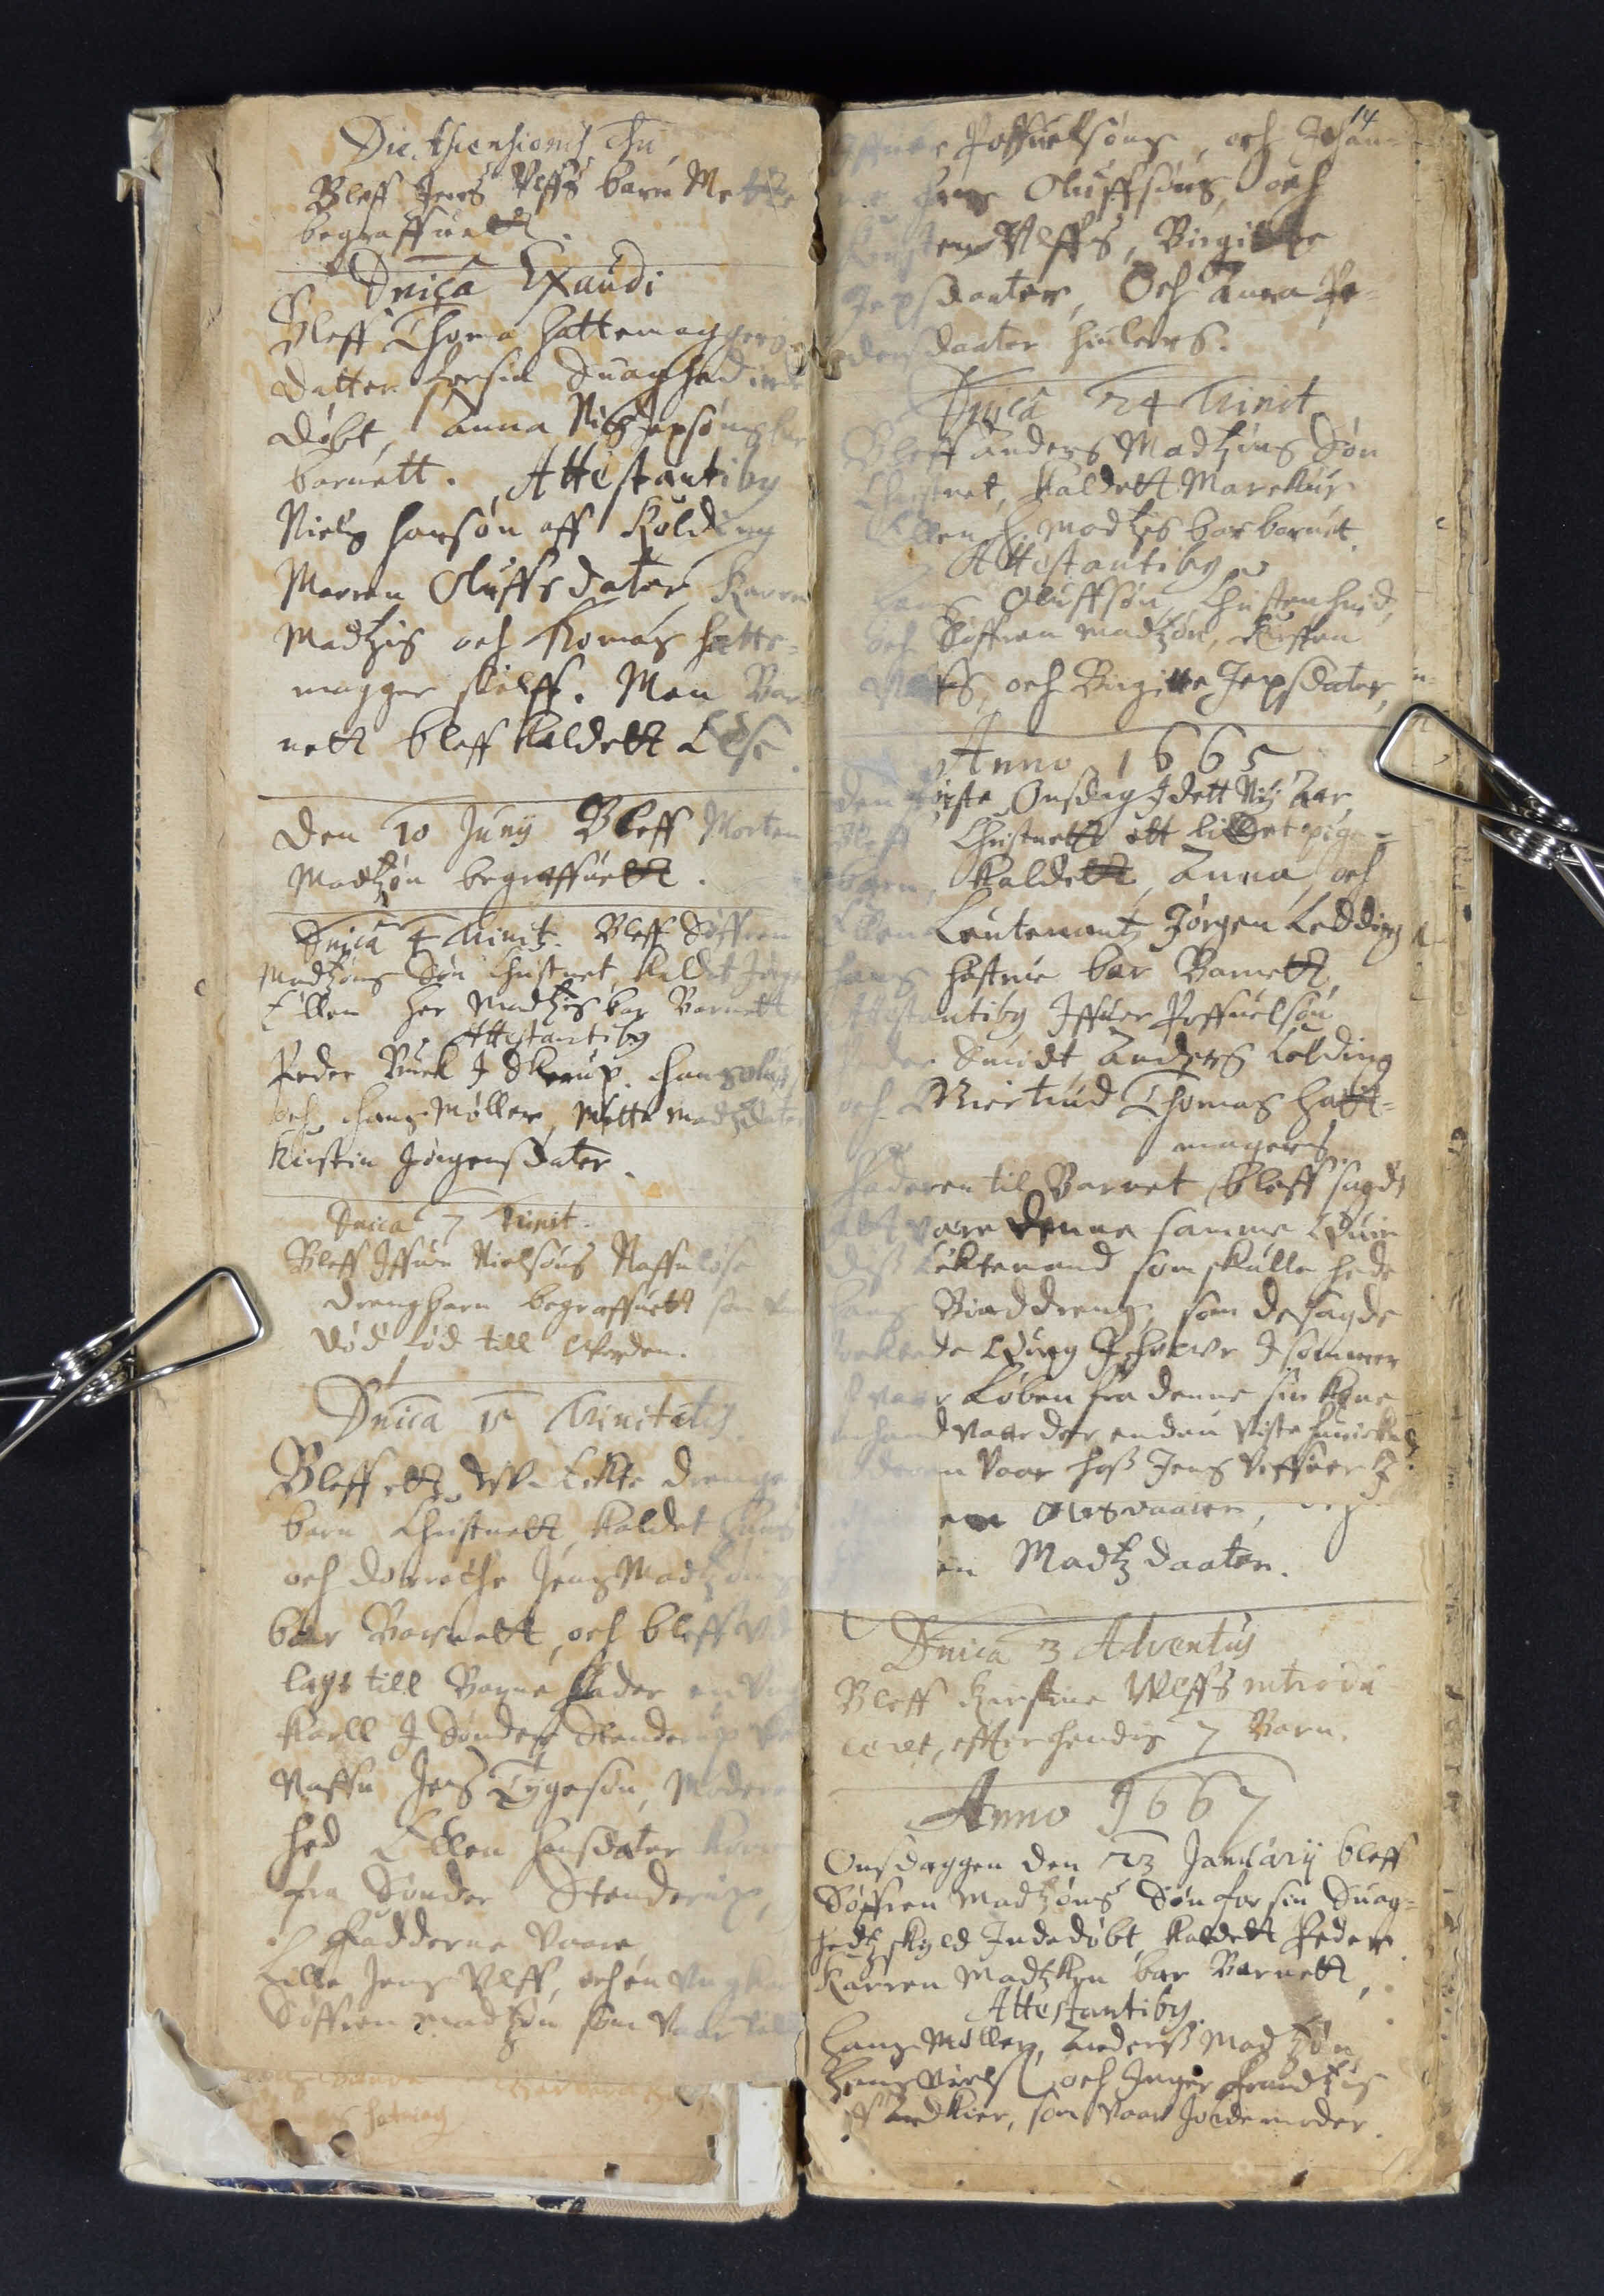Die Ascensionis Chri.
Bleff Jens Vlffs barn Mette
begraffuett
Dnica Exaudi
Bleff Thomas Hattemaggers 
datter for sin Suaghed inde
døbt, Anna Nis Jepsøns bar
barnet. Attestantiby
Niels Hansøn aff Kolding
Marren Oluffsdater Karren
Madtzis och Thomas Hatte¬
magger selff. Men Bar¬
nett bleff kaldett Else.
den 10 Junij. Bleff Morten
Madtzøn begraffuett.
Dnica 4 Trinit. Bleff Søffren
Madtzøns Søn Christnet, kaldet
Ellen Her Madtzis bar Barnett
Attestantiby
Peder Buck I Skerup. Hans
och Hans Møller, Mette Madtzdater
Kirsten Jørgensdater
Dnica 7 Trinit
Bleff Iffuer Nielsøns Naffnløse
Drengebarn baraffuett
død fød till Verden
Dnica 15 Trinitatis
Bleff ett W-eckte Drenge
barn Christnett, kaldet Hans
och Dorrethe Jens Madtz
bar Barnett, och bleff Vd
lagt till Barnefadet en 
karll I Sønder Stenderup 
Naffn Jes Tygesøn, Moderen
hed Ellen Hansdater 
fra Sønder Stenderup,
Fadderne vaare,
Lille Jens Vlff och en vng karl
Søffren Madtzøn som vaar till
14
Iffuer Poffuelsøns, och Johan¬
Hans Oluffsøns och
Kiersten Vlffs, Birgitte
Jepsdaater, och Anna Pe¬
dersdaater Hiulers.
Dnica 24 Trinit
Bleff Anders Madtzøns Søn
Christnet, kaldett Marckus
Ellen H. Madtzis bar barnet
Attestantiby
Hans Oluffsøn, Christen Hvid,
och Søffren Madtzøn. Kiersten
Vlffs och Birgitte Jepsdater
Anno 1665
Den første Onsdag I dett Ny Aar
Bleff Christnett ett lidet pige¬
barn, kaldett, Anna. och
Ellen Liutenant Jørgen Lodding
hans hustrue bar Barnet,
Attestantiby Iffuer Poffuelsøn
Peder Smidt, Anders Colding
och Giertrud Thomas Hatt¬
magers
Faderen til barnet bleff sagdt
att vare denne samme Quin
diss Ecktemand som skulle hede
Hans B##ddreng, som de sagde
Queg I huovr I sommer
var løben fra denne sin kone
hand vaar der endnu viste hun icke,
denn vaar hoss Jens Veffuer I
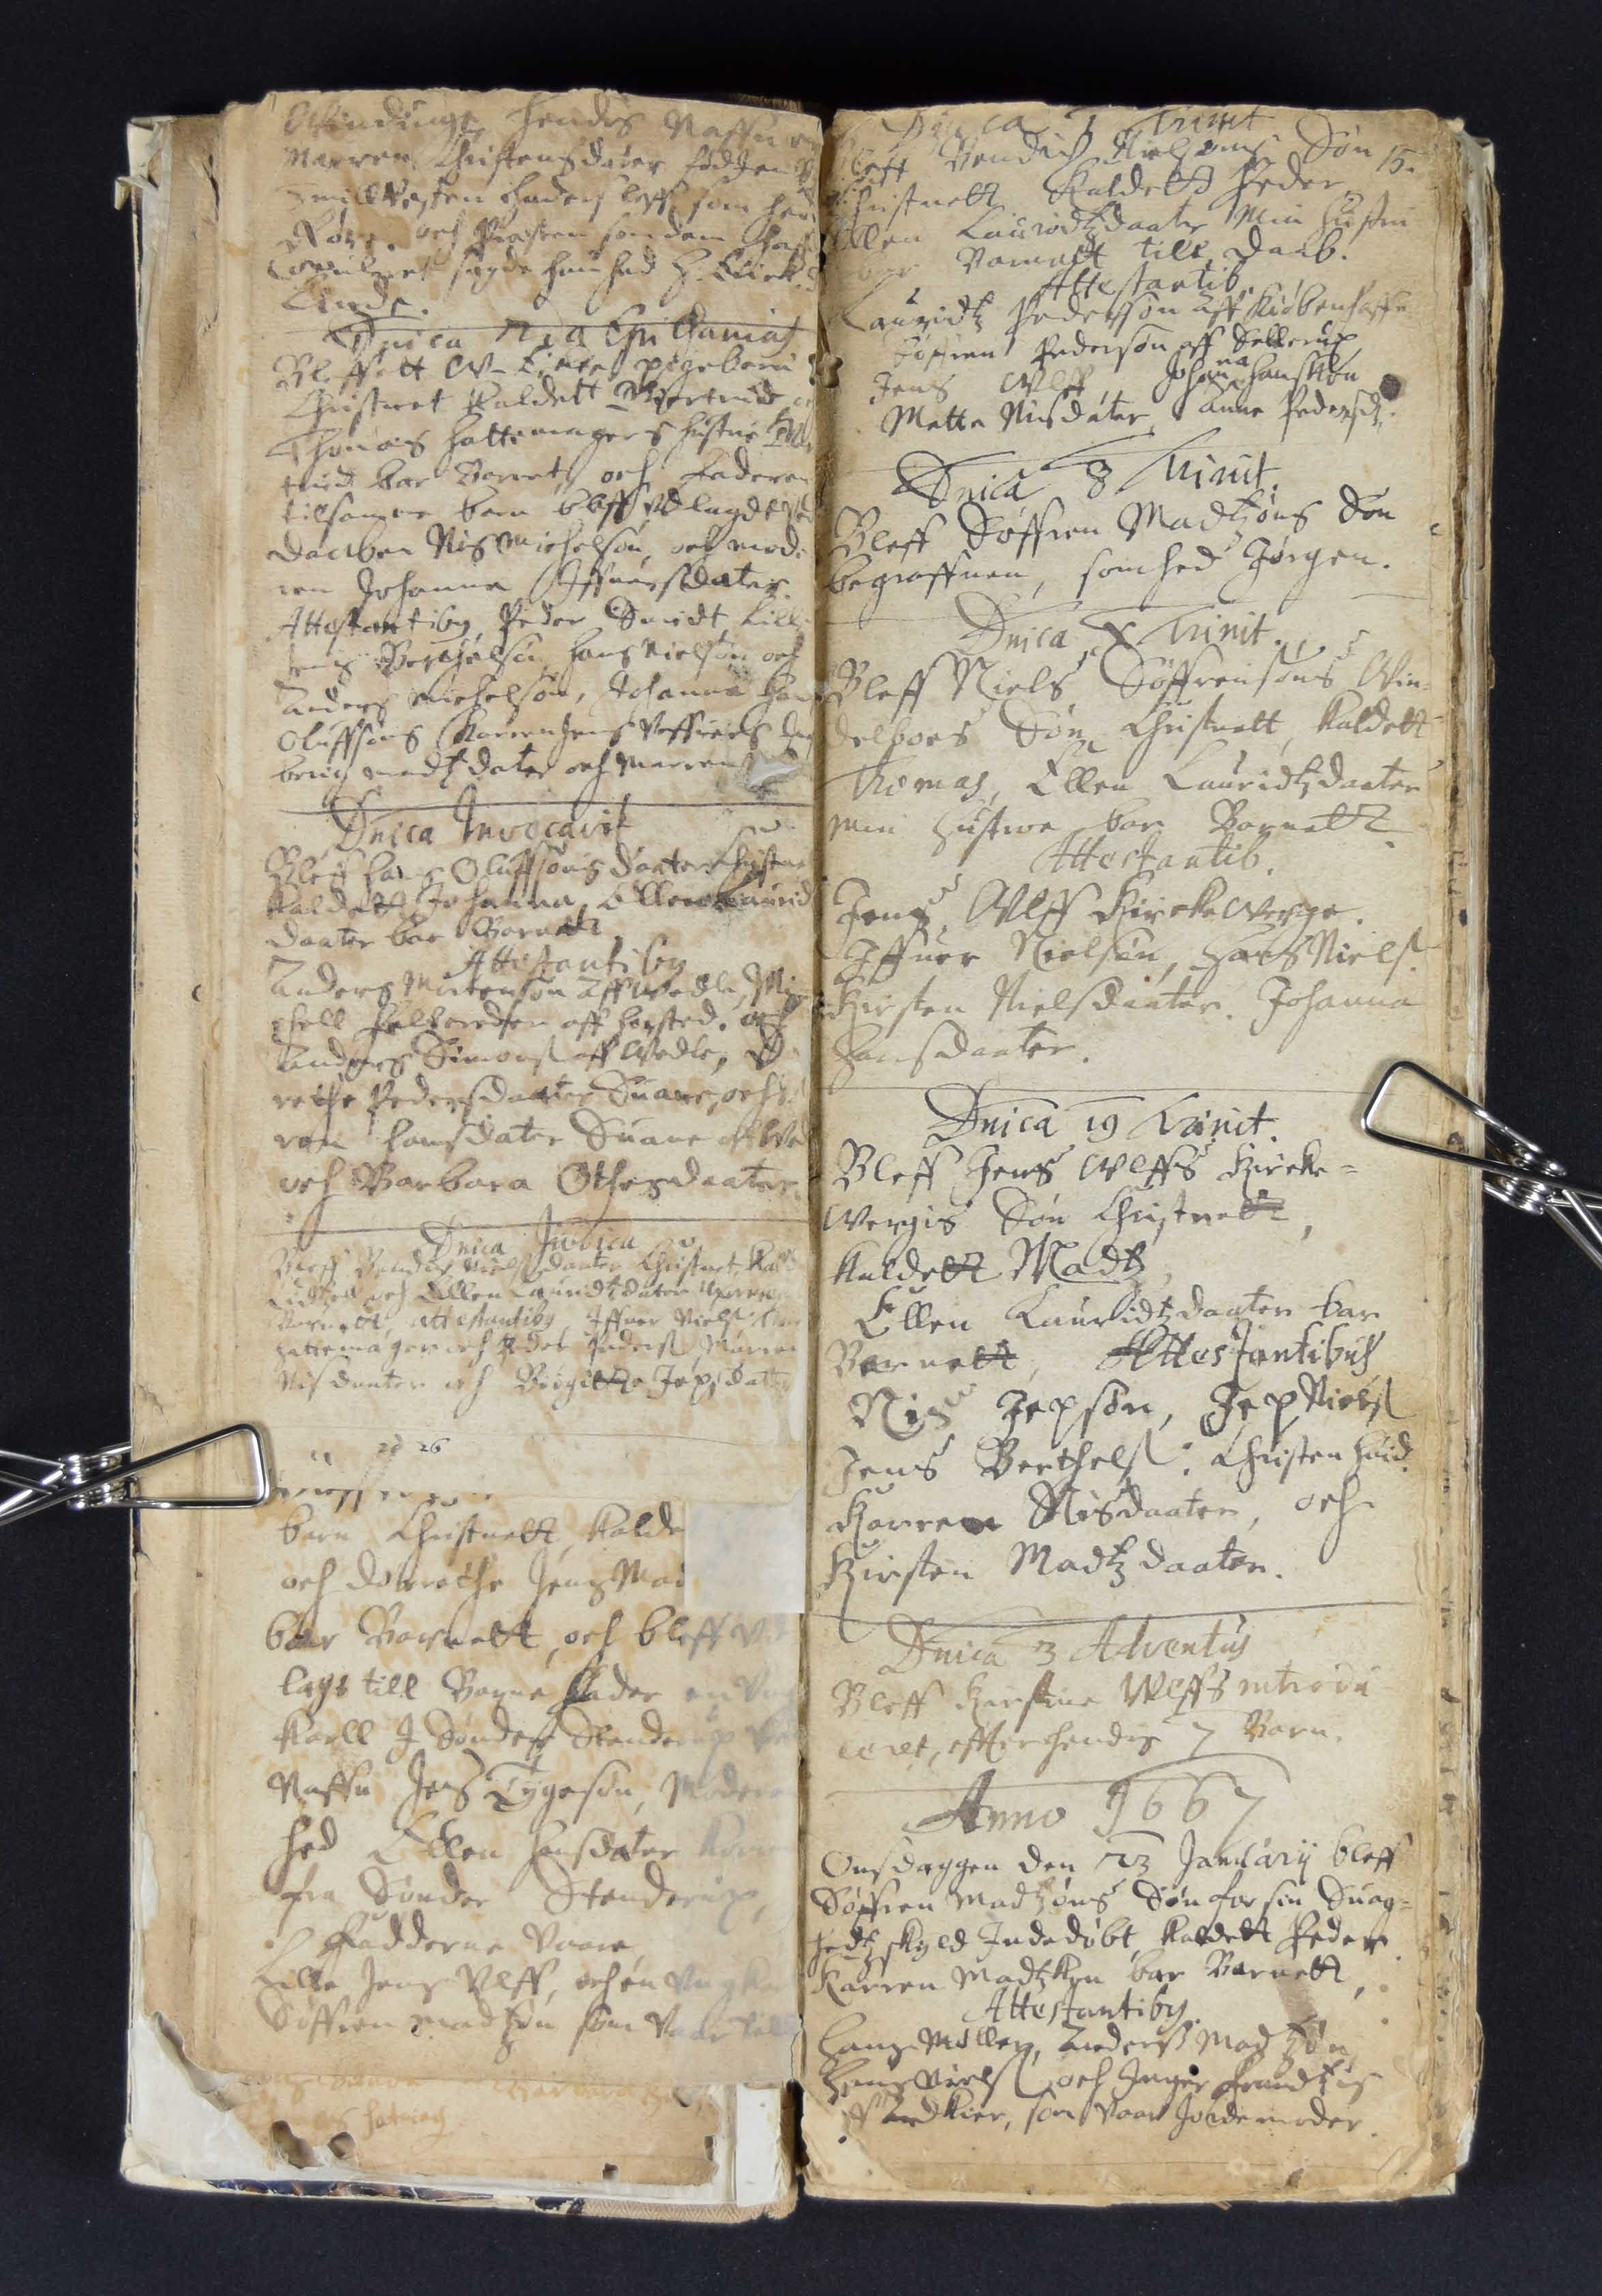Windinge. Hendis naffn va 
Marren Christensdaater, fød I en B 
en Mil Vesten Hadersleff som hede
och Prasten som dem Haff 
Copulerit sagde hun hed H. Erick
Linde.##
Dnica II da Epiphanias.
Bleff ett V-Eike pigebarn
Christnet kaldett Giertrud och
Thomas Hattemagers hustru
trud bar Barnet och faderen
til samme barn bleff Vdlagt ved
Daaben Nis Michelsøn, och mode¬
ren Johanne Iffuersdater.
Attestantiby Peder Smidtz Lille
Jens Berthelsøn, Hans Nielsøn och
Anders Michelsøn, Johanne Hans
Oluffsøns Karren Jens Veffuers.
Madtzdater och Marren
Dnica Invocavit
Bleff Hans Oluffsøns daater Christnet
kaldett Johanna, Ellen Laurid 
daater bar Barnet
Attestantiby
Anders Mortensøn aff Vedle. Mi¬
chell Felbereder aff Horsted. och
Anders Simons aff Vedle, D¬
orthe Pedersdaater Suane, och
ren Hansdater Suane aff Ved 
och Barbara Othesdaater.
Dnica Judica.
Bleff Bendix Niels daater Christnet
Cidtzel, och Ellen Lauridtzdater
Barnett, Attestantiby Iffuer Niels, 
Hattemager och Peder Peders, Maren
Nisdaater och Birgitte Jepsdater.
15.
kaldett Peder
Dnica 1 Trinit
Bleff Bendix Nielsøns Søn
bar Barnet till Daab.
Lauridtzdaater Min hustru
Attestantib
Lauridtz Pederssøn Aff Kiøbenhaffn
Søffren Pedersøn aff Sellerup.
na
Jens Vlff, Johan Hans Kon
Mette Nisdater. Anna Pedersd.
Dnica 8 Trinit.
Bleff Søffren Madtzøns Søn
begraffuen, som hed Jørgen.
Dnica X Trinit.
Bleff Niels Søffrensøn Win
delboes Søn Christnet kaldett
Thomas, Ellen Lauridtzdaater
min Hustru bar Barnett
Attestantil.
Jens Vlff Kirckeverge
Iffuer Nielsøn, Hans Niels
Kirsten Nielsdaater. Johanna
Hansdaater
Dnica 19 Trinit.
Bleff Jens Vlffs Kircke¬
vergis Søn Christnett
kaldett Madtz
Ellen Lauridtzdaater bar
Barnett. Attestantib##
Nis Jepsøn. Jep Niels
Jens Bertels: Christen Hvid
Karren Nisdaater, och
Kirsten Madtzdaater.
Dnica 3 Adventus
Bleff Kirstine Ulffs introdu
ceret efter hendis 7 barn.
Anno 1667
Onsdaggen den 23 Januarij bleff
Søffren Madtzøns Søn for sin Suag¬
hedtz skyld Indedøbt kaldett Peder
Karren Madtz Kon bar Barnett
Attestantiby
Hans Møller, Anderss Madtzøn
Hans Niels och Inger Frandtzis
Andkier, som vaar Jordemoder.